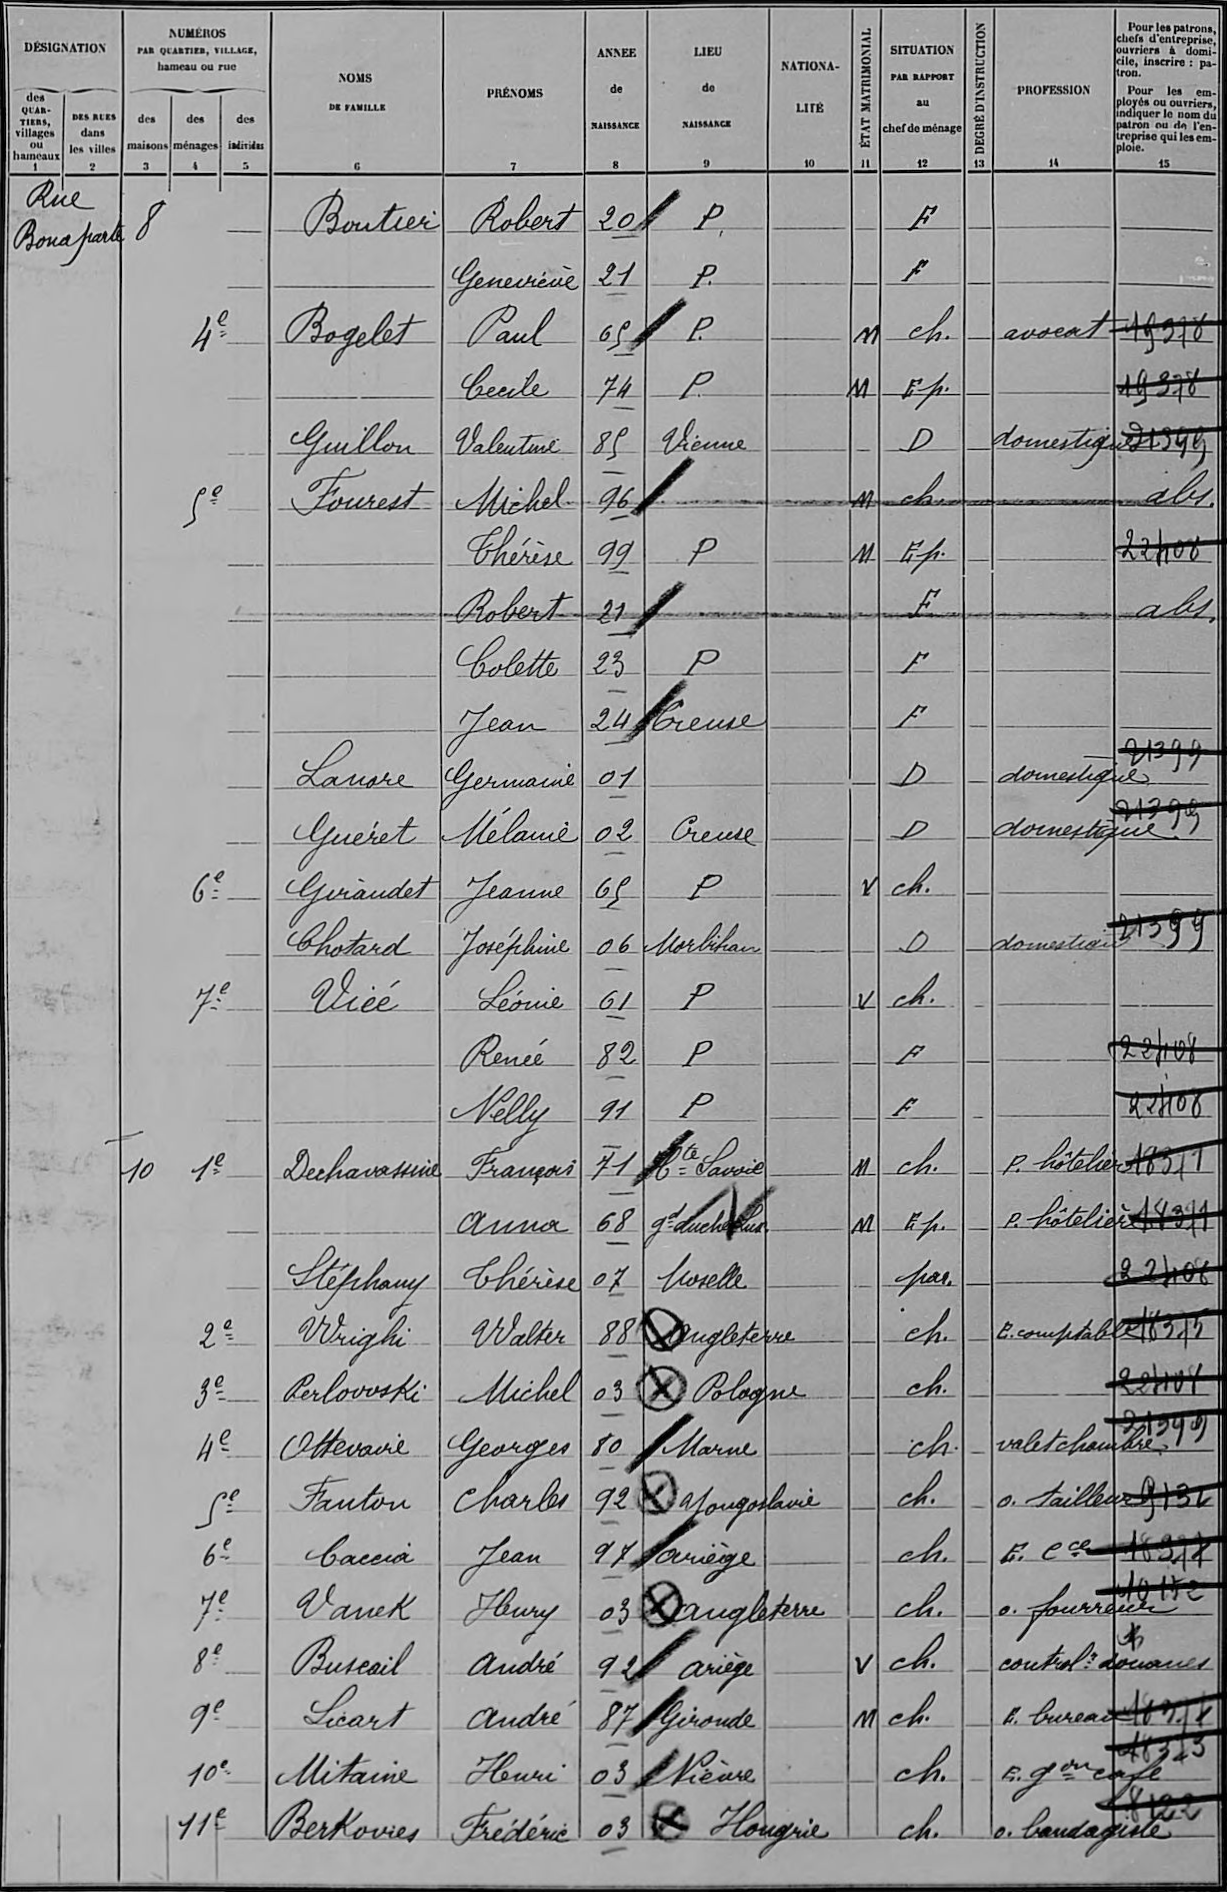Boutier/Robert/20/P/¤/¤/F/¤/¤/¤
¤/Geneviève/21/P /¤/¤/F/¤/¤/¤
Bogelet/Paul/65/P /¤/M/ch /¤/avocat/19378
¤/Cecile/74/P /¤/M/Ep /¤/¤/19378
Guillon/Valentine/85/Vienne/¤/¤/D/¤/domestique/21399
Fourest/Michel/96/¤/¤/M/ch /¤/¤/abs
¤/Thérèse/99/P/¤/M/Ep /¤/¤/22408
¤/Robert/21/¤/¤/¤/F/¤/¤/abs
¤/Colette/23/P/¤/¤/F/¤/¤/¤
¤/Jean/24/Creuse/¤/¤/F/¤/¤/¤
Lanore/Germaine/01/¤/¤/¤/D/¤/domestique/21399
Guéret/M2lanie/02/Creuse/¤/¤/D/¤/domestique/?21399
Giraudet/Jeanne/65/P/¤/V/ch /¤/¤/¤
Chotard/Joséphine/06/Morbihan/¤/¤/D/¤/domesique/21399
Viée/Léonie/61/P/¤/v/Ch /¤/¤/¤
¤/Renée/82/P/¤/¤/F/¤/¤/22408
¤/Nelly/91/P/¤/¤/F/¤/¤/22408
Dechavassine/François/71/Hte Savoie/¤/M/ch /¤/P hôtelier/18371
¤/Anna/68/Gd duché Lux/¤/M/Ep /¤/P hôtelière/18371
Stéphany/Thérèse/07/Moselle/¤/¤/par /¤/¤/22408
Wrigli/Walter/88/Angleterre/¤/¤/ch /¤/E comptable/18375
Perlowski/Michel/03/Pologne/¤/¤/ch /¤/¤/22408
Ottevaire/Georges/80/Marne/¤/¤/Ch /¤/valet chambre/21399
Fauton/Charles/92/Yougoslavie/¤/¤/ch /¤/o tailleur/9132
Caccia/Jean/97/Ariège/¤/¤/ch /¤/E Cce/18377
Vanek/Henry/03/Angleterre/¤/¤/ch /¤/o fourreur/10152
Buascail/André/92/Ariège/¤/V/ch /¤/coutrole douanes/¤
Sicart/André/87/Gironde/¤/M/ch /¤/E bureau/18377
Mitaine/Henri/0/Nièvre/¤/¤/ch /¤/E gon café/¤
Berkovies/Frédéric/03/Hongrie/¤/¤/ch /¤/o bandagiste/?8122
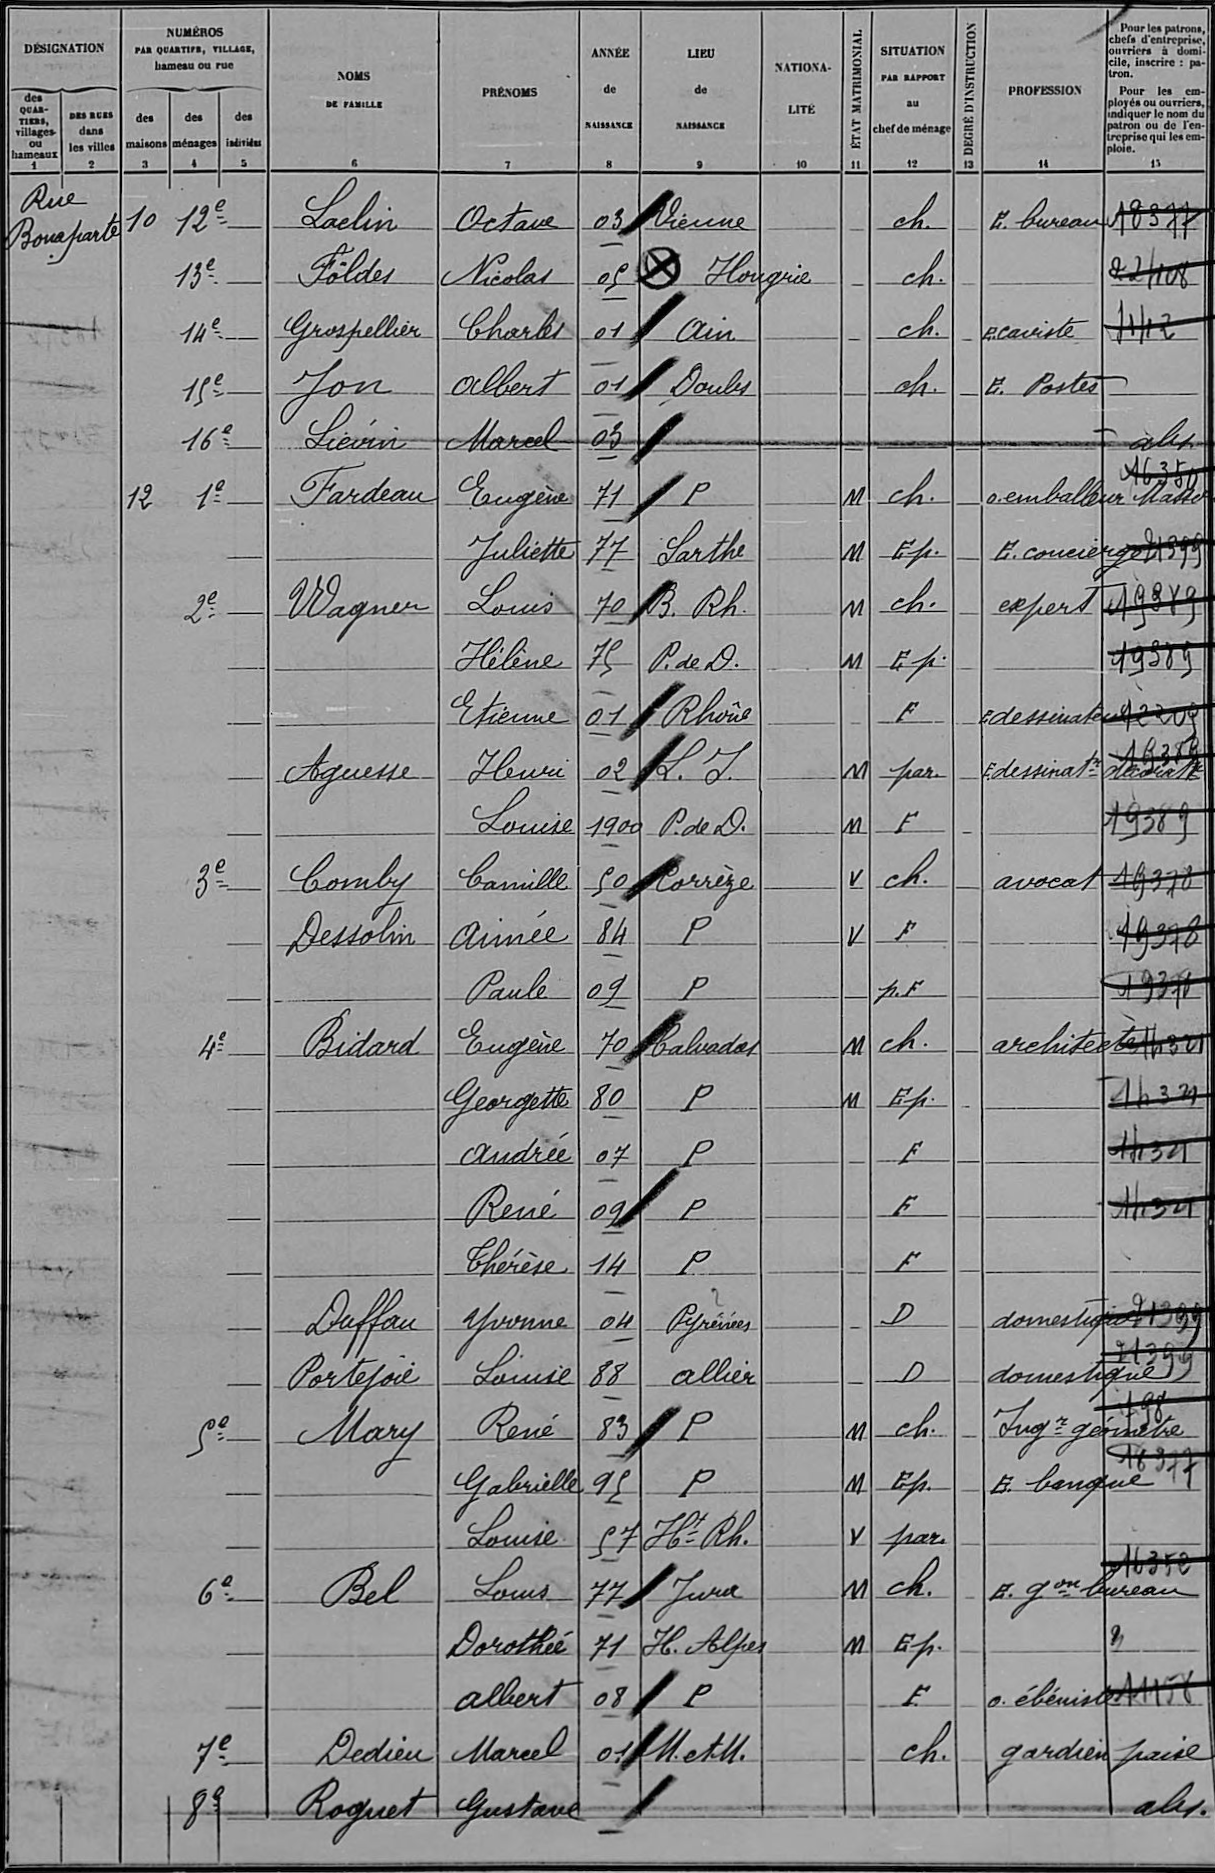Laclin/octave/03/Vienne/¤/¤/ch /¤/E bureau/18377
Fôldes/Nicolas/05/Hongrie/¤/¤/ch /¤/¤/22408
Grospellier/Charles/01/Ain/¤/¤/ch /¤/E caviste/442
Jon/Albert/01/Doubs/¤/¤/ch /¤/E Postes/¤
Liévin/Marcel/03/¤/¤/¤/¤/¤/¤/abs
Fardeau/Eugène/71/P/¤/M/ch /¤/o emballeur Masset/16350
¤/Juliette/77/Sarthe/¤/M/Ep /¤/E concierge/21399
Wagner/Louis/70/B Rh /¤/M/ch /¤/expert/19389
¤/Hélène/75/P de D /¤/M/Ep/¤/¤/19389
¤/Etienne/01/Rhône/¤/¤/F/¤/E dessinateur/12209
Aguesse/Henri/02/L I /¤/M/par/¤/E dessinateur decorat/?19389
¤/Louise/1900/P de D /¤/M/F/¤/¤/19389
Comby/Aimée/84/P/¤/V/F/¤/¤/19378
Dessolin/Aimée/84/P/¤/V/F/¤/¤/19378
¤/Paule/09/P/¤/¤/p F/¤/¤/19378
Bidard/Eugène/70/Calvados/¤/M/ch /¤/architecte/14321
¤/Georgette/80/P/¤/M/Ep/¤/¤/15321
¤/Andrée/07/P/¤/¤/F/¤/¤/14321
¤/René/09/P/¤/¤/F/¤/¤/14321
¤/Thérèse/14/P/¤/¤/F/¤/¤/¤
Duffau/Yvonne/04/Pyrénées/¤/¤/D/¤/domestique/21399
Portejoie/Louise/88/Allier/¤/¤/D/¤/domestique/21399
Mary/René/83/P/¤/M/ch /¤/Ingr géomètre/798
¤/Gabrielle/95/P/¤/M/Ep /¤/E banque/?18377
¤/Louise/57/Ht Rh /¤/V/par /¤/¤/¤
Bel/Louis/77/Jura/¤/M/ch /¤/E gon burau/?16352
¤/Dorothée/71/H Alpes/¤/M/Ep /¤/¤/¤
¤/Albert/08/P/¤/¤/E/¤/o ébéniste/11158
Dedieu/Marcel/01/M et M /¤/¤/ch /¤/gardien paix/¤
Roguet/Gustave/¤/¤/¤/¤/¤/¤/¤/abs